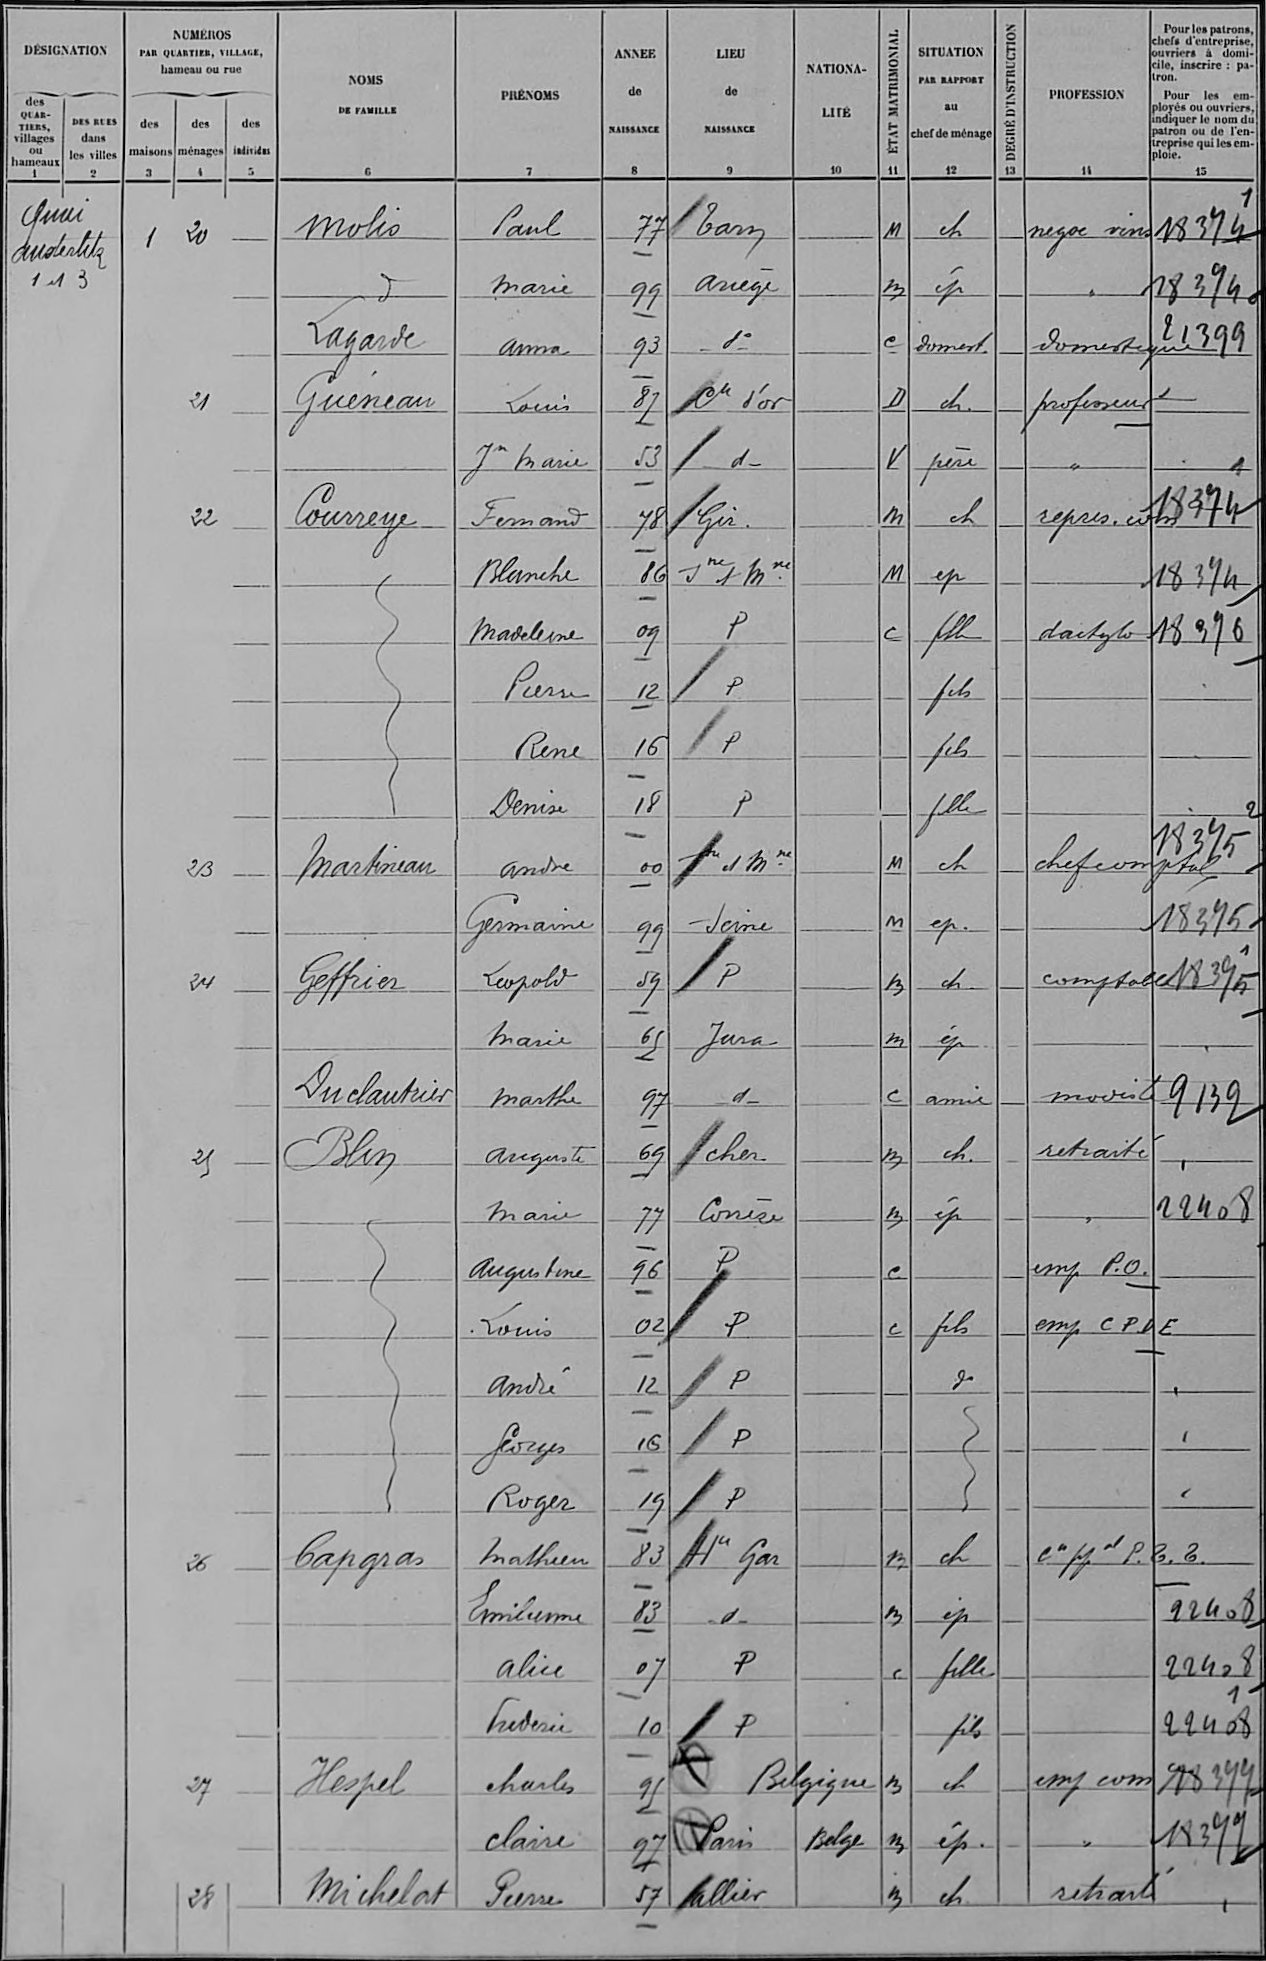Molis/Paul/77/Tarn/¤/M/ch/¤/negoc vins/18374 1
d/Marie/99/Ariège/¤/m/ép/¤/¤/18374
Lagarde/Anna/93/d°/¤/c/domest /¤/domestique/21399
Guéneau/Louis/81/Ct d'or/¤/D/ch /¤/professeur/¤
¤/Jn Marie/53/d/¤/V/père/¤/¤/¤
Courreye/fernand/78/Gir /¤/m/ch/¤/repres com/18374
¤/Blanche/86/Sne t Mne/¤/M/ep/¤/¤/18374
¤/Madeleine/09/P/¤/C/fille/¤/dactylo/18376
¤/Pierre/12/P/¤/¤/fils/¤/¤/¤
¤/René/16/P/¤/¤/fils/¤/¤/¤
¤/Denise/18/P/¤/¤/fille/¤/¤/2
Martineau/Andre/00/Sne et Mne/¤/M/ch/¤/chef comptab/?18375
¤/Germaine/99/Seine/¤/M/ep /¤/¤/18375
Geffrier/Leopold/59/P/¤/m/ch /¤/comptable/18395 1
¤/Marie/65/Jura/¤/m/ép/¤/¤/¤
Duclautrier/Marthe/97/d/¤/c/amie/¤/modiste/9132
Blin/Auguste/69/cher/¤/m/ch /¤/retraité/¤
¤/Marie/77/Corrèze/¤/m/ép/¤/¤/22408
¤/Augustine/96/P/¤/c/¤/¤/imp P O /¤
¤/Louis/02/P/¤/c/fils/¤/emp C P D E/¤
¤/André/12/P/¤/¤/d/¤/¤/¤
¤/Georges/16/P/¤/¤/¤/¤/¤/¤
¤/Roger/19/P/¤/¤/¤/¤/¤/¤
Capgras/Mathieu/83/Hte Gar/¤/m/ch/¤/c ppal /P T T
¤/Emilienne/83/d/¤/m/ép/¤/¤/22408
¤/alice/07/P/¤/c/fille/¤/¤/22408 1
¤/Frederic/10/P/¤/¤/fils/¤/¤/22408
Hespel/Charles/95/Belgique/¤/m/ch/¤/emp com/18 399
¤/Claire/97/Paris/Belge/m/ép /¤/¤/18399
Michelat/Pierre/57/allier/¤/m/ch/¤/retraité/¤
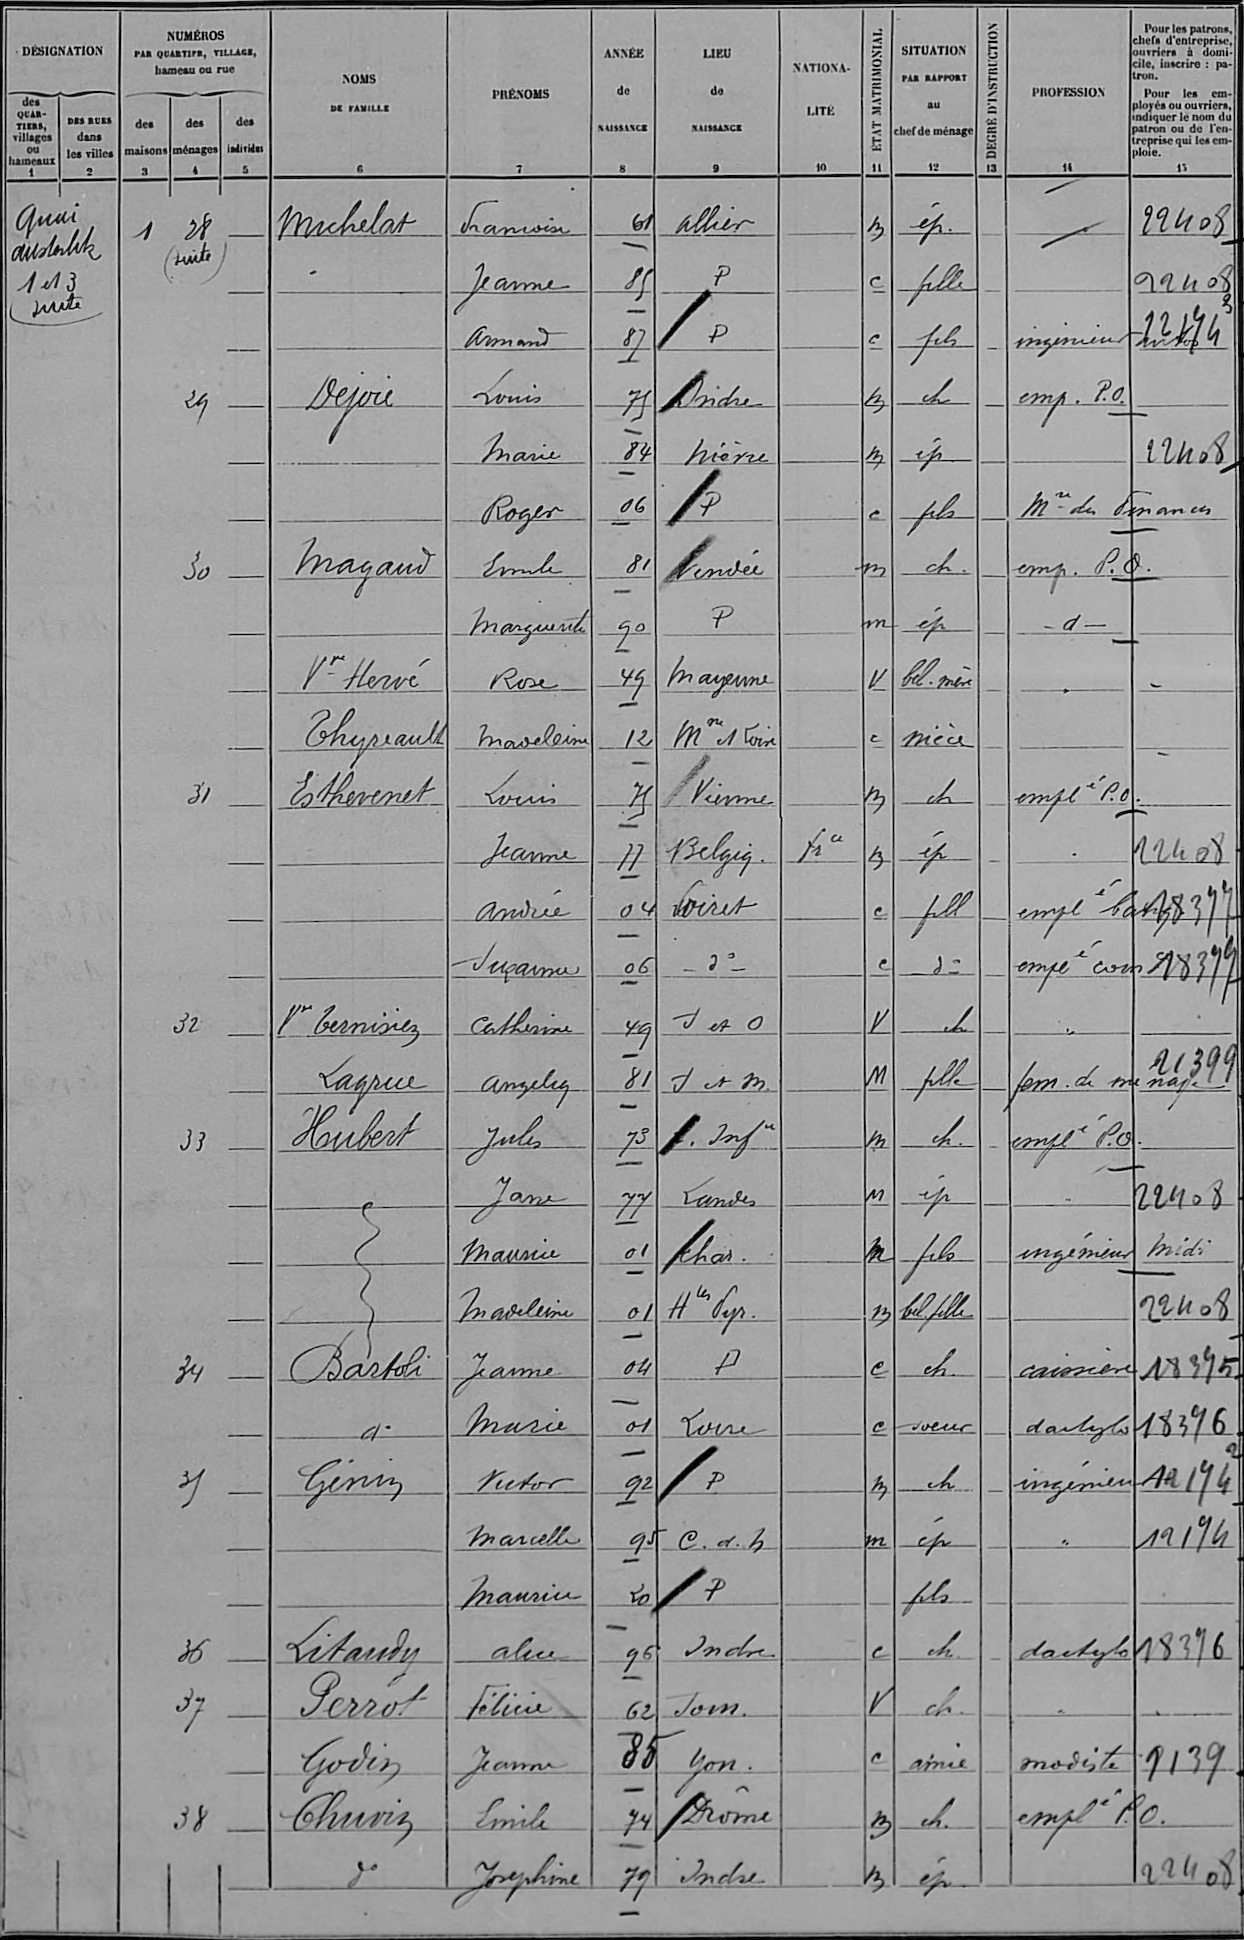Michelat/Francoise/61/Allier/¤/m/ép /¤/¤/22408
¤/Jeanne/85/P/¤/C/fille/¤/¤/22408
¤/Armand/87/P/¤/C/fils/¤/ingénieur autos/?22174
Dejoie/Louis/75/Indre/¤/m/ch/¤/emp P O /¤
¤/Marie/84/Nièvre/¤/m/ép /¤/¤/22408
¤/Roger/06/P/¤/c/fils/¤/Mre des Finances/¤
Magaud/Emile/81/Vendée/¤/m/ch /¤/emp P O /¤
¤/Marguerite/90/P/¤/m/ép/¤/d/¤
Vve Hervé/Rose/49/Mayenne/¤/V/bel mère/¤/¤/¤
Thyreault/Madeleine/12/Mne et Loire/¤/c/nièce/¤/¤/¤
Esthevenet/Louis/75/Vienne/¤/m/ch/¤/empl P O /¤
¤/Jeanne/77/Belgiq/Frce/m/ép/¤/¤/22408
¤/Andrée/04/Loiret/¤/c/fill/¤/emplé banq/?18377
¤/Suzanne/06/d°/¤/c/d°/¤/emplé com/18377
Vve Bernisiez/Catherine/49/S et O/¤/V/ch/¤/¤/¤
Lagrue/Angeliq/81/s et m /¤/M/fille/¤/fem de menage/21399
Hubert/Jules/73/c Infre/¤/m/ch /¤/emplé P O /¤
¤/Jane/77/Landes/¤/M/ép/¤/¤/22408
¤/maurice/01/char /¤/m/fils/¤/ingénieur midi/¤
¤/Madeleine/01/Htes Pyr/¤/M/bel fille/¤/¤/22408
Bartoli/Jeanne/04/P/¤/C/ch /¤/caissière/18375
d°/Marie/01/Loire/¤/c/soeur/¤/dactylo/18376
Génin/Victor/92/P/¤/m/ch/¤/ingénieur/12174 2
¤/Marcelle/95/C d N/¤/m/ép/¤/¤/19174
¤/Maurice/20/P/¤/¤/fils/¤/¤/¤
Litaudy/Alice/96/Indre/¤/c/ch/¤/dactylo/18376
Perrot/Felicie/62/Tarn/¤/V/ch/¤/¤/¤
Godin/Jeanne/85/Yon /¤/c/amie/¤/modiste/9139
Chuvin/Emilie/74/Drome/¤/M/ch /¤/emplé P O /¤
d°/Josephine/79/Indre/¤/M/ép /¤/¤/22408